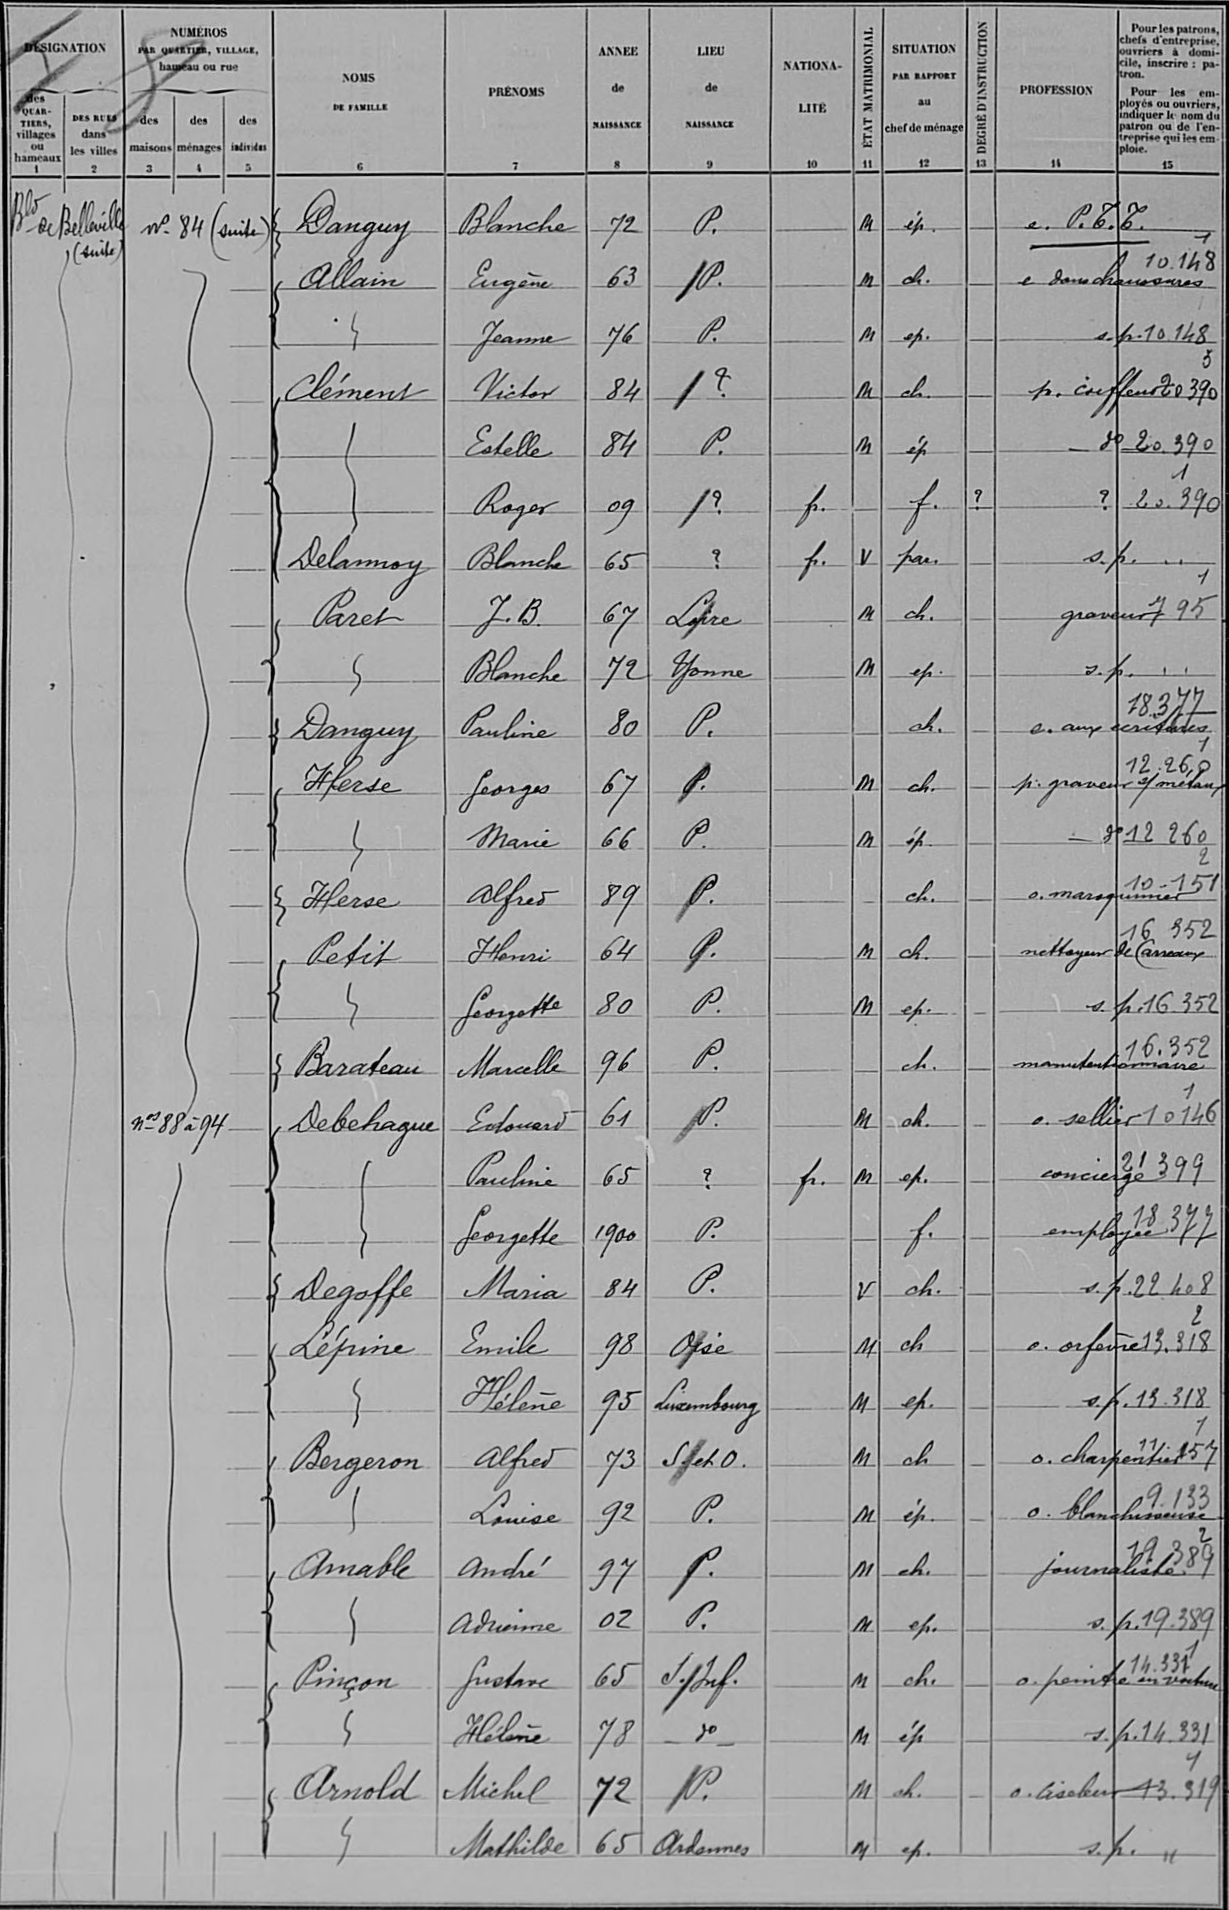Danguy/Blanche/72/P /¤/M/ép /¤/e P T T/¤
Allain/Eugène/63/P /¤/M/ch /¤/e dans chaussures/¤
¤/Jeanne/76/P /¤/M/ep /¤/s p /10148
Clément/Victor/84/¤/¤/M/ch /¤/p coiffeur/20390
¤/Estelle/84/P /¤/M/ép/¤/d°/20390
¤/Roger/09/¤/fr /¤/f /¤/¤/20390
Delamoy/Blanche/65/¤/f /V/par /¤/s p/¤
Paret/J B /67/Loire/¤/M/ch /¤/graveur/795
¤/Blanche/72/Yonne/¤/M/ep /¤/s p /¤
Danguy/Pauline/80/P /¤/¤/ch /¤/e aux écriture/¤
Herse/Georges/67/P /¤/M/ch /¤/p graveur s métaux/¤
¤/Marie/66/P /¤/M/ép /¤/d°/12260
Herse/Alfred/89/P /¤/¤/ch /¤/o maroquinier/10151
Petit/Henri/64/P /¤/M/ch /¤/nettoyeur de carreaux/¤
¤/Georgette/80/P /¤/M/ep /¤/s p /16352
Barateau/Marcelle/96/P /¤/¤/ch /¤/manutentionnaire/16352
Debehague/Edouard/61/P /¤/M/ch /¤/o sellier/10146
¤/Pauline/65/¤/fr /M/ep /¤/concierge/21399
¤/Georgette/1900/P /¤/¤/f /¤/employée/18377
Degoffe/Maria/84/P /¤/V/ch /¤/s p /22408
Lépine/Emile/98/Oise/¤/M/ch/¤/o orfèvre/13318
¤/Hélène/95/Luxembourg/¤/M/ep /¤/s p /13318
Bergeron/Alfred/73/S et O /¤/M/ch/¤/o charpentier/¤
¤/Louise/92/P /¤/M/ép /¤/o blanchisseuse/¤
Amable/André/97/P /¤/M/ch /¤/journaliste/¤
¤/Adrienne/02/P /¤/M/ep /¤/s p /19389
Pinçon/Gustave/65/S Inf /¤/M/ch /¤/o peintre en voiture/¤
¤/Hélène/78/d°/¤/M/ép/¤/s p /14331
Arnold/Michel/72/P /¤/M/ch /¤/o ciseleur/13319
¤/Mathilde/65/Ardennes/¤/M/ep /¤/s p /¤
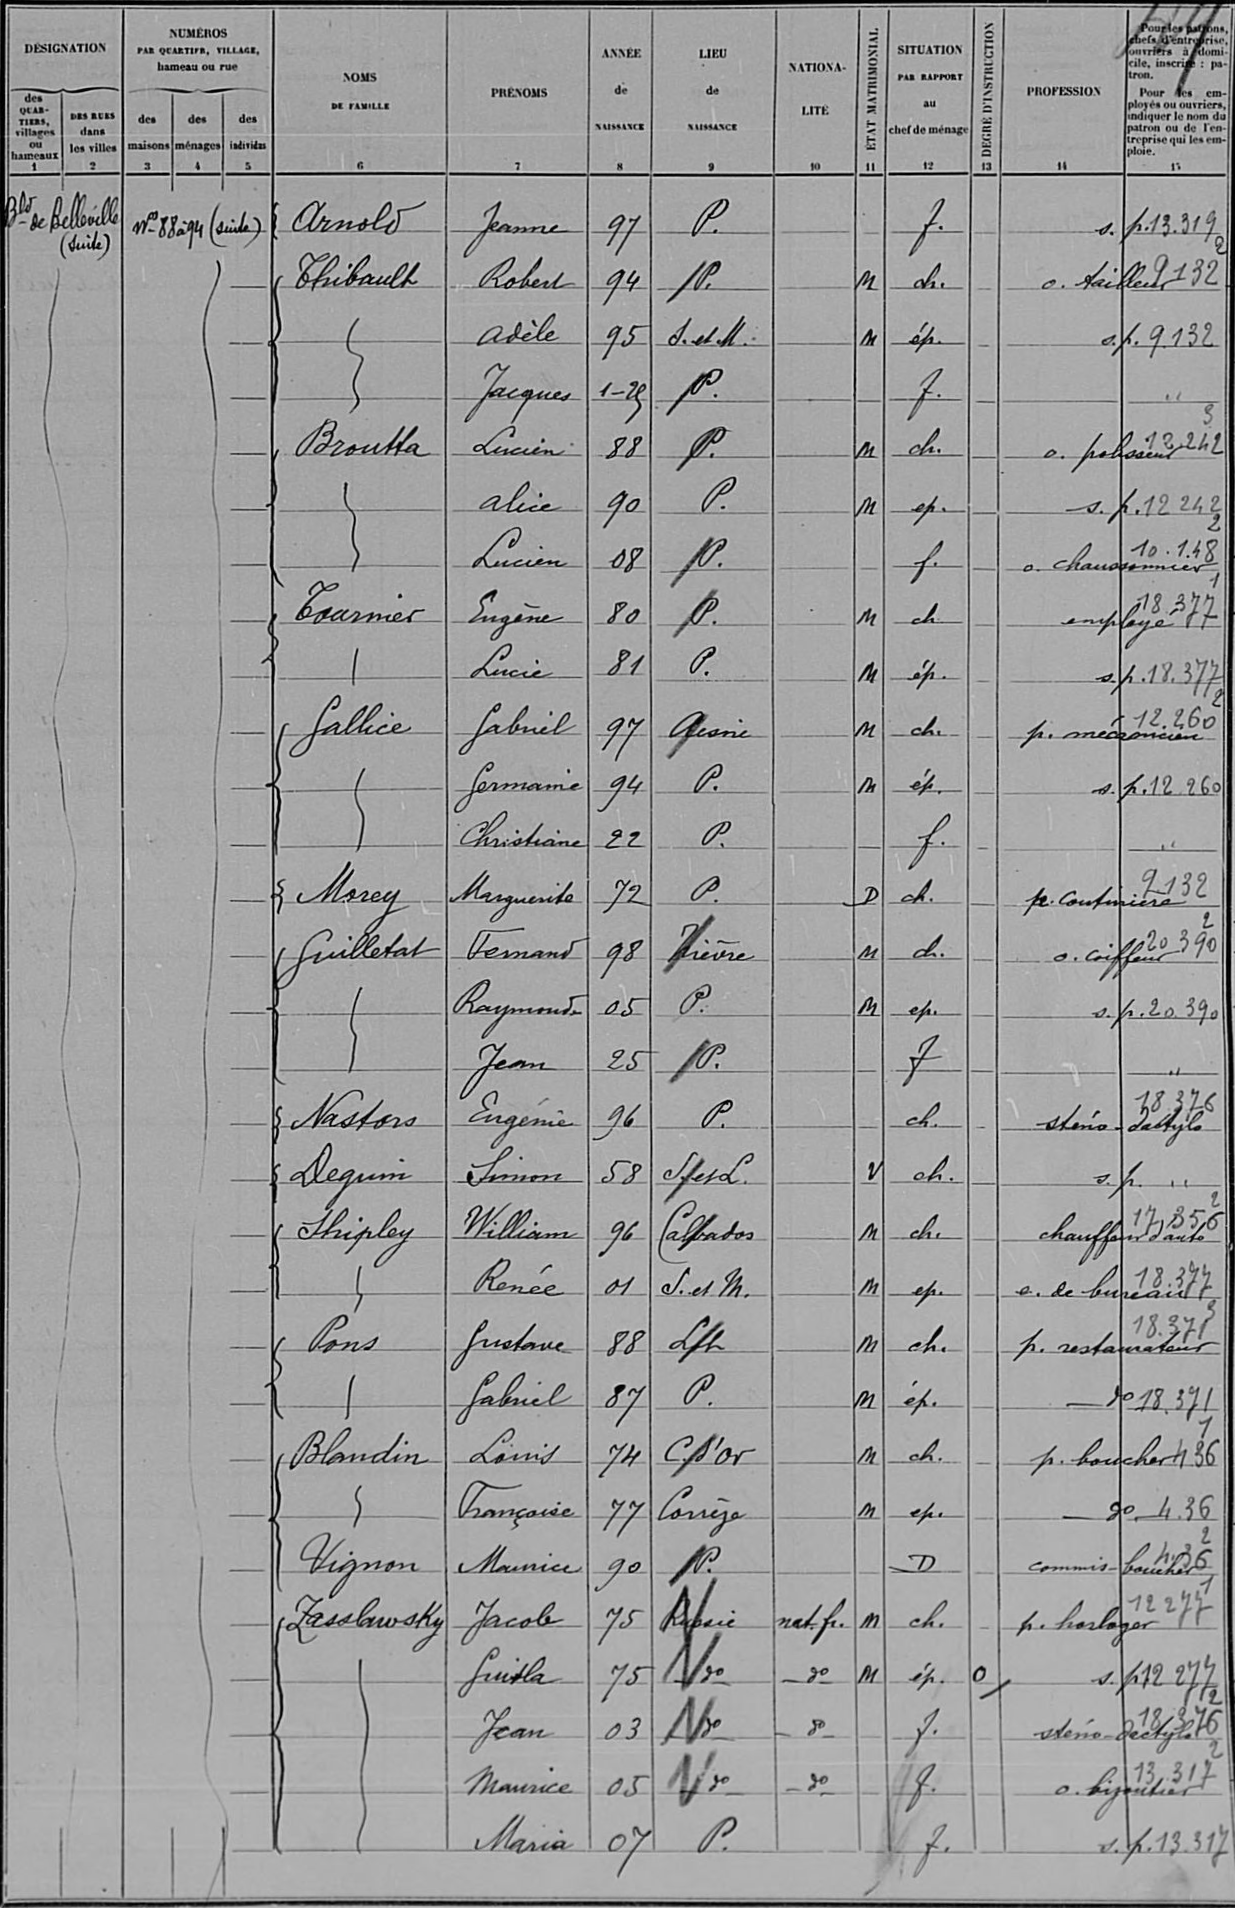Arnold/Jeanne/97/P /¤/¤/f /¤/s p /13319
Thibault/Robert/94/P /¤/M/ch /¤/o tailleur/9132
¤/Adèle/95/S et M /¤/M/ép /¤/s p /9132
¤/Jacques/1 25/P /¤/¤/f /¤/¤/¤
Broussa/Lucien/88/P /¤/M/ch /¤/o polisseur/12242
¤/alice/90/P /¤/M/ep /¤/s p /12242
¤/Lucien/08/P /¤/¤/f /¤/o chaussonnier/¤
Tournier/Eugène/80/P /¤/M/ch/¤/employé/¤
¤/Lucie/81/P /¤/M/ép /¤/s p /18377
Gallice/Gabriel/97/Aisne/¤/M/ch /¤/p mécanicien/¤
¤/Germaine/94/P /¤/M/ép /¤/s p /12260
¤/Christiane/22/P /¤/¤/f /¤/¤/¤
Morey/Marguerite/72/P /¤/D/ch /¤/p couturière/¤
Guilletat/Fernand/98/Nièvre/¤/M/ch /¤/o coiffeur/¤
¤/Raymonde/05/P /¤/M/ep /¤/s p /20390
¤/Jean/25/P /¤/¤/f/¤/¤/¤
Nastors/Euégnie/96/P /¤/¤/ch /¤/sténo-dactylo/¤
Deguin/Simon/58/S et L /¤/V/ch /¤/s p /¤
Shipley/William/96/Calvados/¤/M/ch /¤/chauffeur d'auto/¤
¤/Renée/01/S et M /¤/M/ep /¤/e de bureau/18377
Pons/Gustave/88/Lot/¤/M/ch /¤/p restaurateur/¤
¤/Gabriel/87/P /¤/M/ép /¤/d°/18371
Blandin/Louis/74/C d'Or/¤/M/ch /¤/p boucher/436
¤/Françoise/77/Corrèze/¤/M/ep /¤/d°/436
Vignon/Maurice/90/P /¤/¤/D/¤/commis boucher/436
Zasslawsky/Jacob/75/Russie/nat fr /m/ch /¤/p horloger/¤
¤/Guisla/75/d°/d°/M/ép/0/s p /12277
¤/Jean/03/d°/d°/¤/f /¤/sténo-dactylo/18376
¤/Maurice/05/d°/d°/¤/f /¤/o bijoutier/13317
¤/Maria/07/P /¤/¤/f /¤/s p /13317
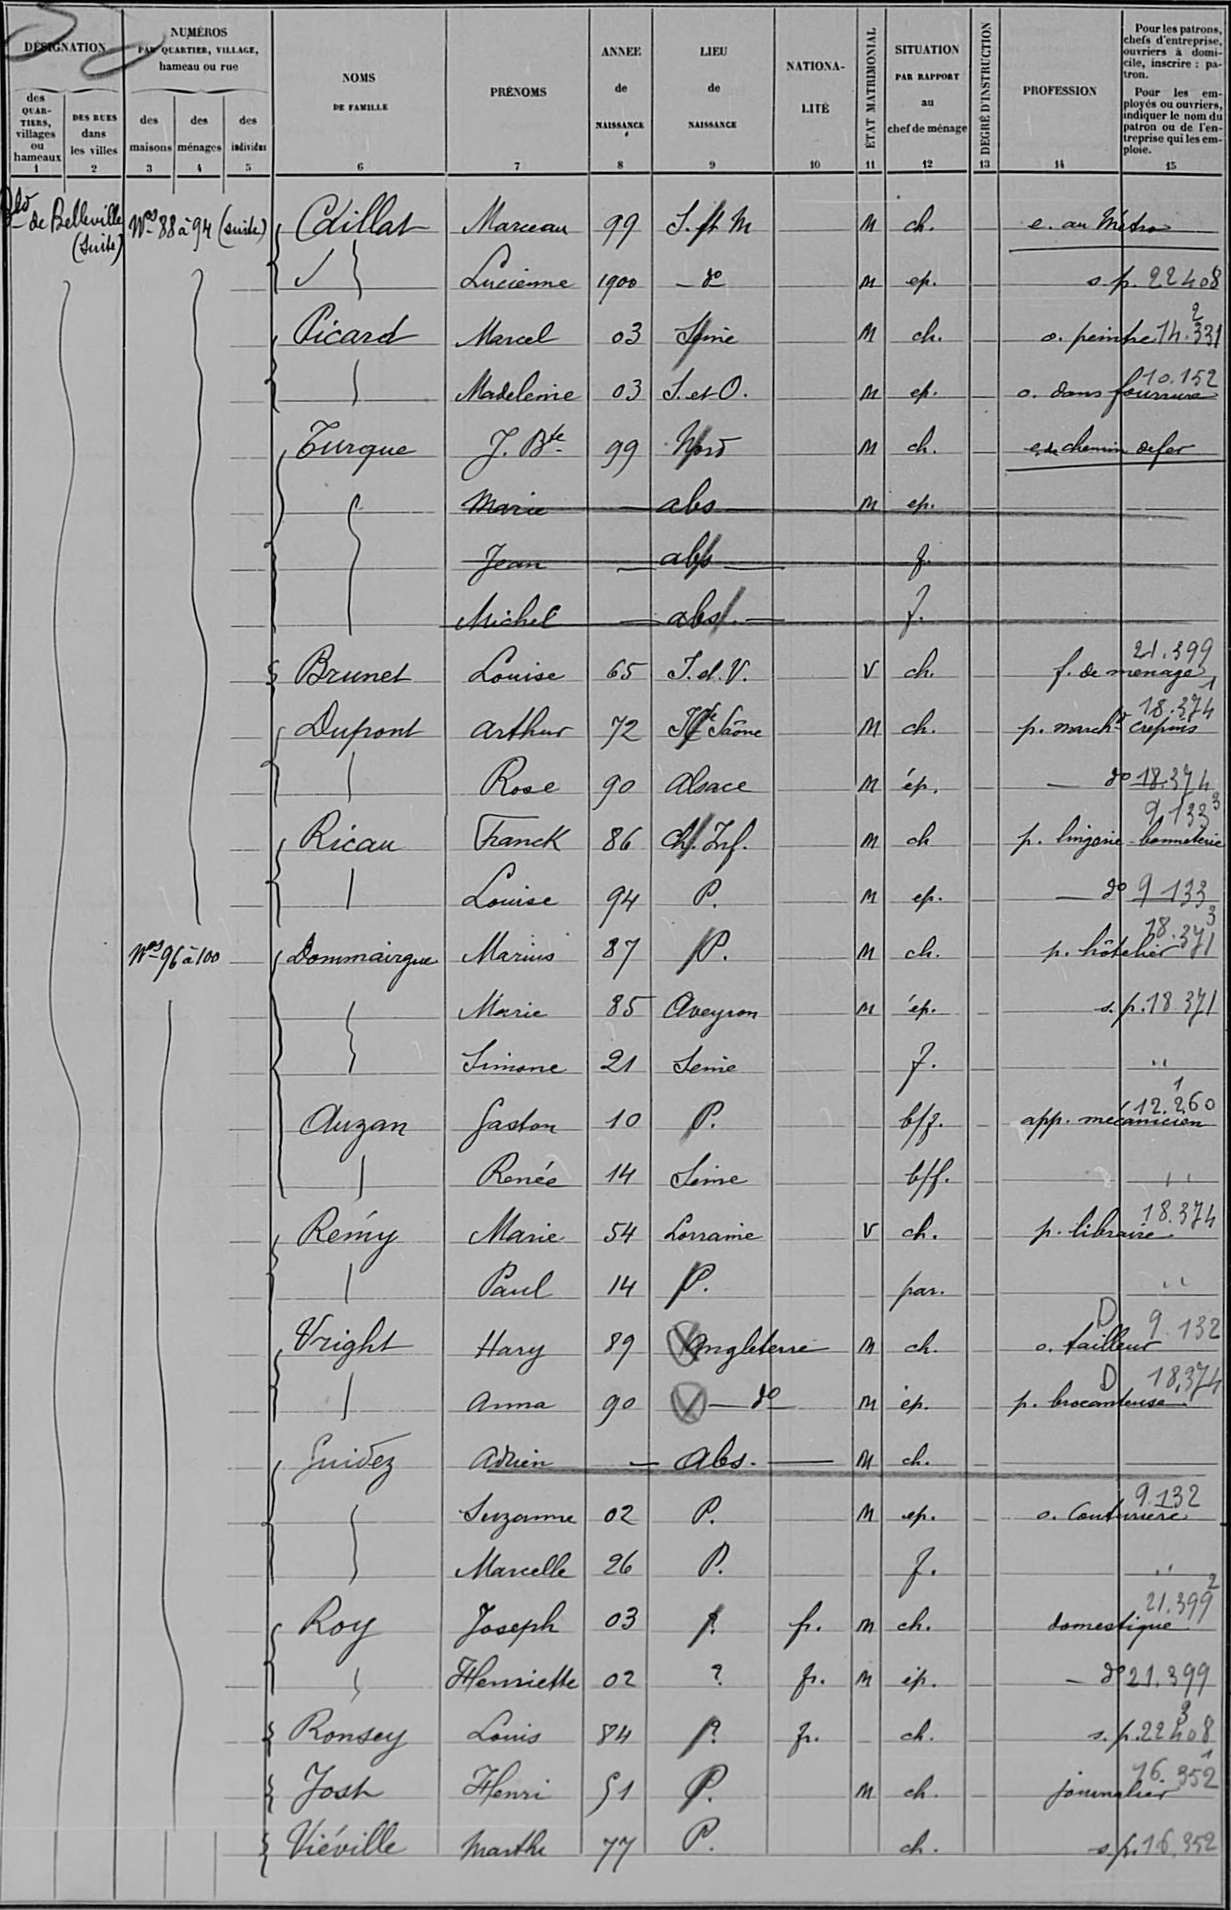Caillat/Marceau/99/S et M/¤/M/ch /¤/e au Métro/¤
¤/Lucienne/1900/d°/¤/M/ep /¤/s p /22408
Picard/Marcel/03/Seine/¤/M/ch /¤/o peintre/14331
¤/Madeleine/03/S et O /¤/M/ep /¤/o dans fourrure/¤
Turque/J Bte/99/Nord/¤/M/ch /¤/e de chemin de fer/¤
¤/Marie/¤/abs/¤/M/ep /¤/¤/¤
¤/Jean/¤/abs/¤/¤/f /¤/¤/¤
¤/Michel/¤/abs/¤/¤/f /¤/¤/¤
Brunet/Louise/65/I et V /¤/V/ch /¤/f de ménage/¤
Dupont/Arthur/72/Hte Saône/¤/M/ch /¤/p marchd crepons/¤
¤/Rose/90/Alsace/¤/M/ép /¤/d°/18374
Ricau/Franck/86/Ch Inf /¤/M/ch/¤/p lingerie-bonneterie/¤
¤/Louise/94/P /¤/M/ep /¤/d°/9133
Dommairgue/Marius/87/P /¤/M/ch /¤/p hôtelier/¤
¤/Marie/85/Aveyron/¤/M/ép /¤/s p /18371
¤/Simone/21/Seine/¤/¤/f /¤/¤/¤
Auzan/Gaston/10/P /¤/¤/b:f /¤/app mécanicien/12260
¤/Renée/14/Seine/¤/¤/b f /¤/¤/¤
Rémy/Marie/54/Lorraine/¤/V/ch /¤/p libraire/18374
¤/Paul/14/P /¤/¤/par /¤/¤/¤
Vright/Hary/89/Angleterre/¤/M/ch /¤/o tailleur/D 9132
¤/Anna/90/d°/¤/M/ép /¤/p brocanteuse/¤
Guidez/Adrien/¤/Abs /¤/M/ch /¤/¤/¤
¤/Suzanne/02/P /¤/M/ep /¤/o couturière/¤
¤/Marcelle/26/P /¤/¤/f /¤/¤/¤
Roy/Joseph/03/¤/fr /M/ch /¤/domestique/¤
¤/Henriette/02/¤/fr /M/ép /¤/d°/21399
Ronsey/Louis/84/¤/fr /¤/ch /¤/s p /22408
Jost/Henri/51/P /¤/M/ch /¤/journalier/16352
Viéville/Marthe/77/P /¤/¤/ch /¤/s p /16352